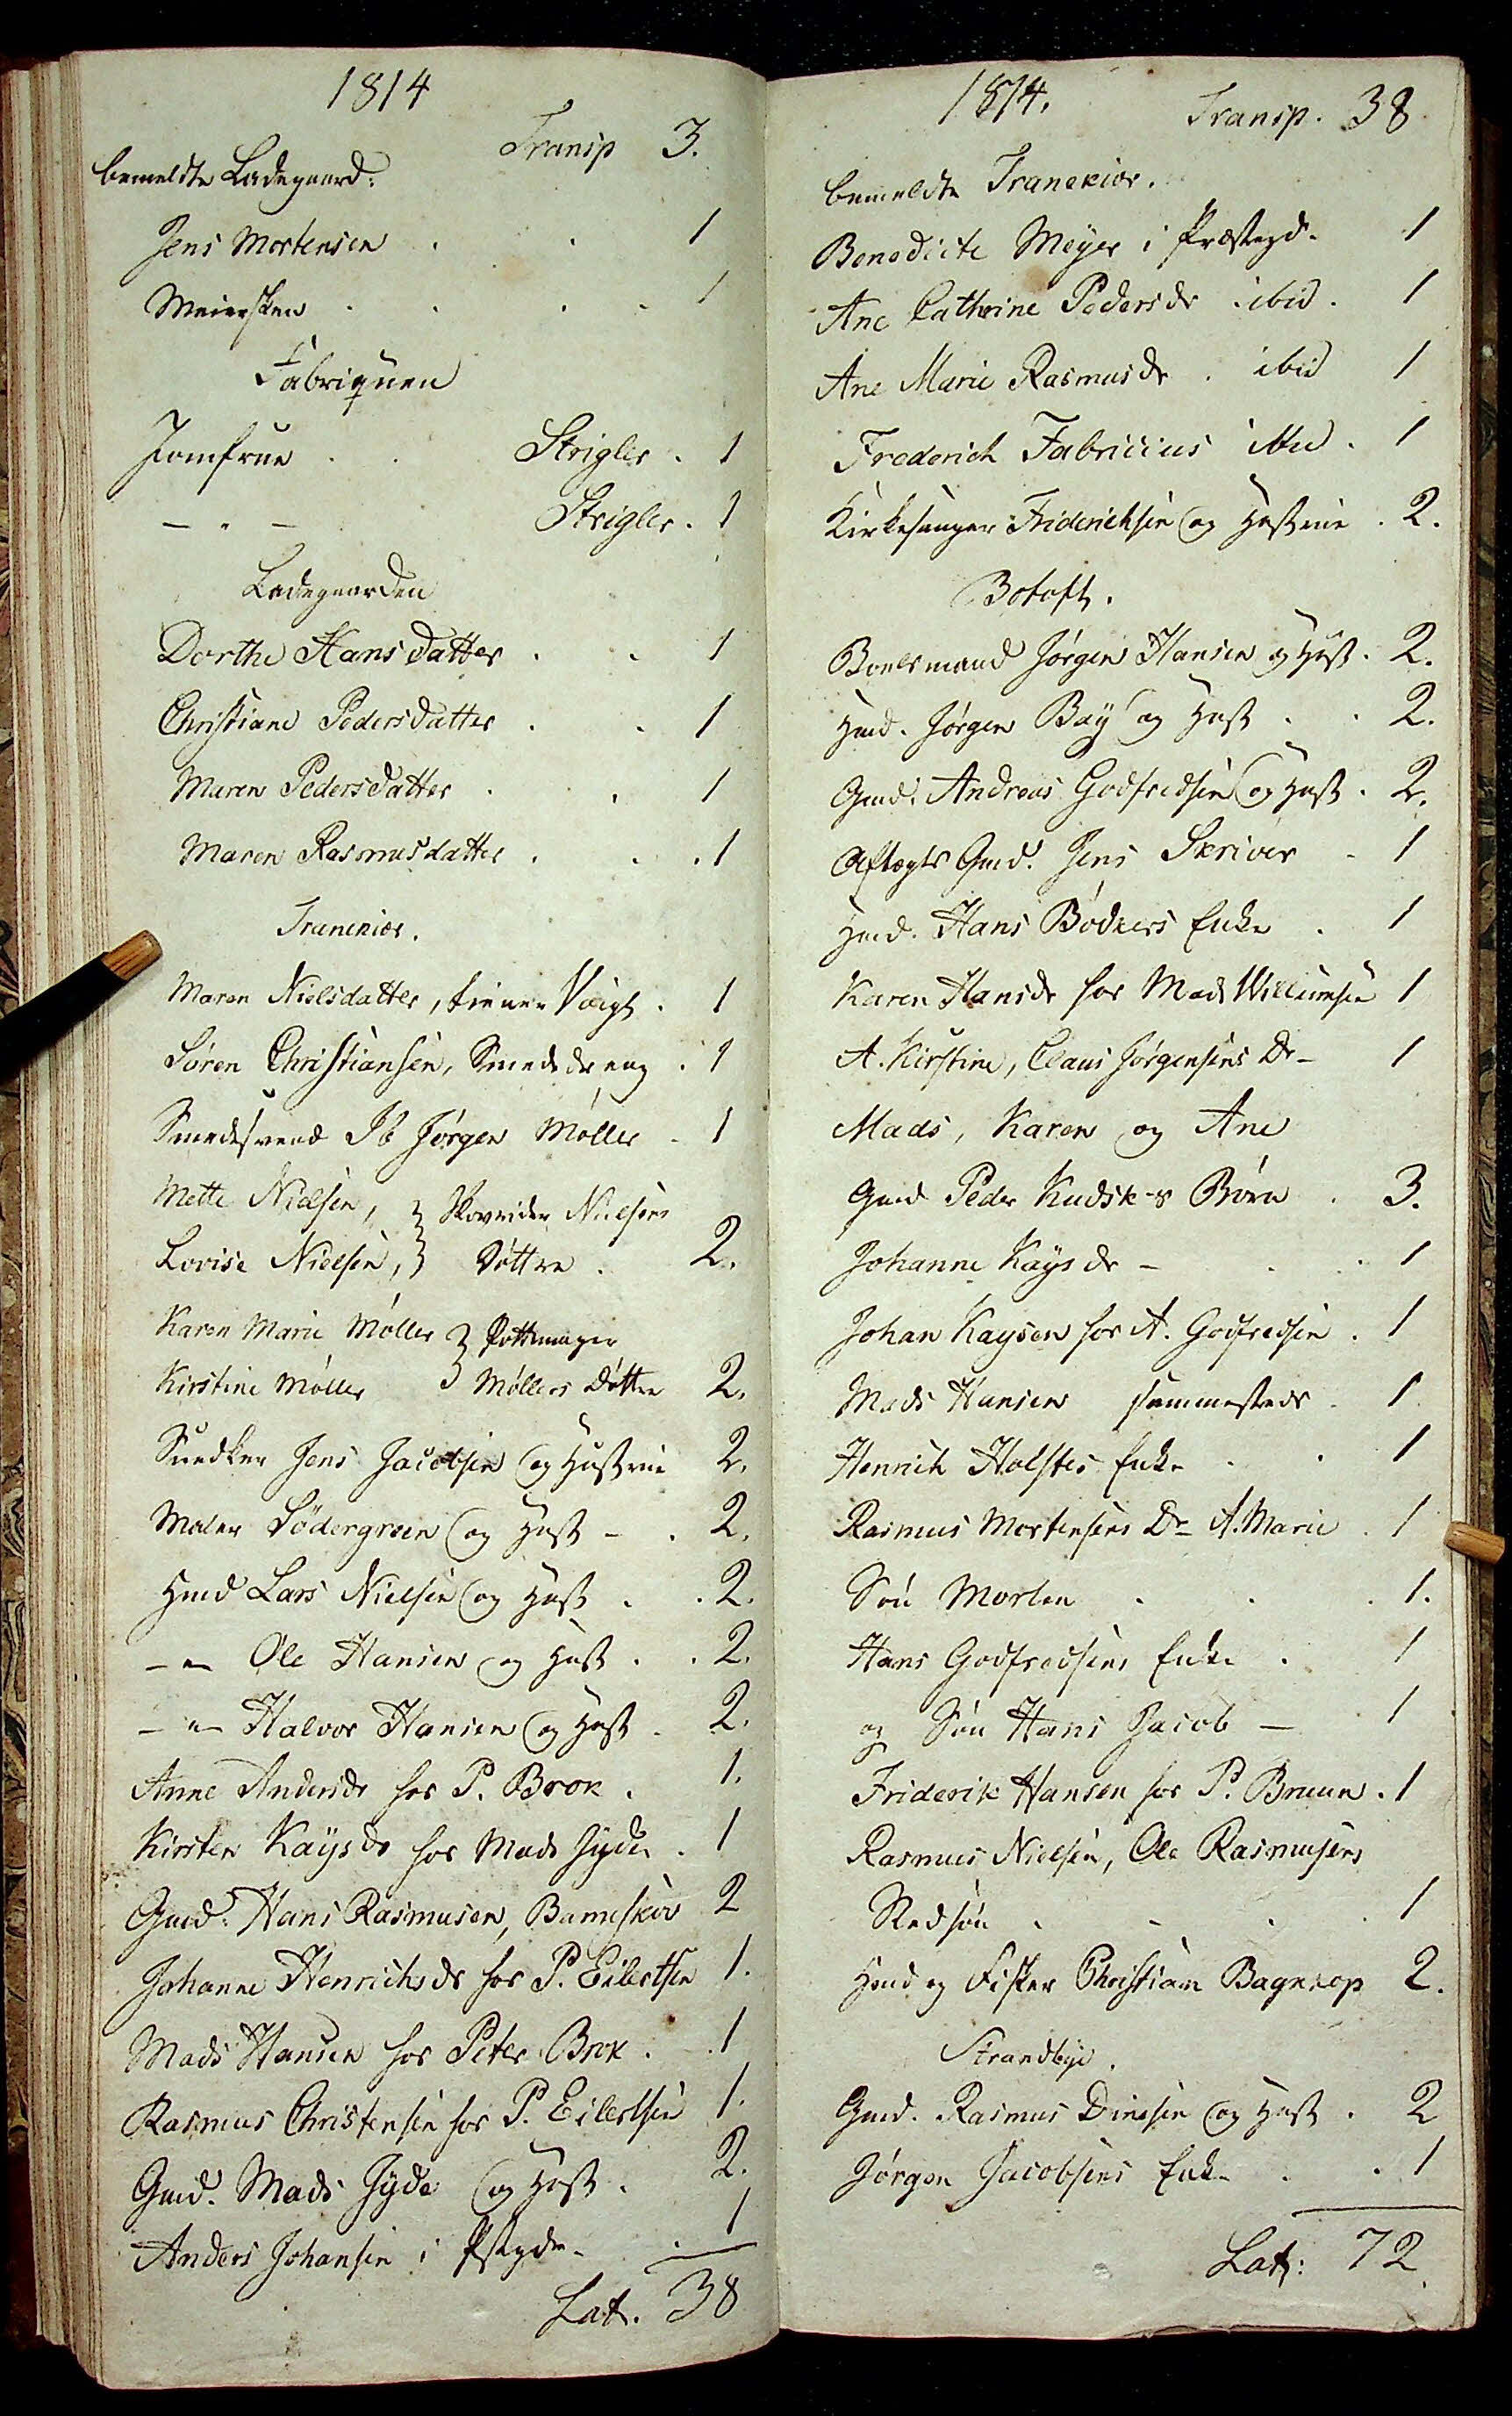1814
Transp 3.
bemeldte Ladegaard:
Jens Mortensen . . 1
Meiersken . . . . 1
Fabriquen
Jomfrue . . Strigler . 1
-"- . Strigler . 1
Ladegaarden
Dorthe Hansdatter . . 1
Christiane Pedersdatter . . 1
Maren Pedersdatter . . . 1
Maren Rasmusdatter . . . 1
Tranekiær
Maren Nielsdatter, tienner Voigt . 1
Søren Christiansen, Smeddreng . 1
Smedsvend Ib Jørgen Møller . 1
Mette Nielsen,
Lovise Nielsen,
Skovrider Nielsens
Døttre . 2.
Karen Marie Møller
Pottemager
Kirstine Møller Møllers Døttre 2.
Snedker Jens Jacobsen og Hustrue 2.
Maler Sødergreen og Hust - . 2.
Hmd Lars Nielsen og Hust . . 2.
-"- Ole Hansen og Hust .  . 2.
-"- Halvor Hansen og Hust . 2.
Anne Andersdr hos P. Brok . 1.
Kirsten Kaysdr hos Mads Jyde . 1
Gmd: Hans Rasmusen Bameskov 2
Johanne Henrichsdr hos P. Eilertsen 1.
Mads Hansen hos Peter Brok . . 1
Rasmus Christensen hos P. Eilertsen 1.
Gud Mads Jyde og Hust . 2
Anders Johansen i Pstgdr . .1
Lat. 38
1814.
Transp. 38
bemeldte Tranekiær.
Bendicte Meyer i Præstedg . 1
Ane Cathrine Pedersdr ibid . 1
Anne Marie Rasmusdr . ibid 1
Frederich Fabricius ibid . 1
Kirkesanger Friderichsen og Hustrue . 2.
Botoft.
Boelsmand Jørgen Hansen og Hust . 2.
Hmd. Jørgen Bay og Hust . . 2.
Gmd Anders Godfredsen og Hust . 2.
Aftægts Gmd. Jens Skriver . 1
Hmd. Hans Bødkers Enke . 1
Karen Hansdr hos Mads Willumsen 1
A. Kirstine, Claus Jørgensens Dr - 1
Mads, Karen og Ane
Gmd Peder Kudsk-s Børn . 3.
Johanne Kaysdr - . . 1
Johan Kaysen hos Godfredsen . 1
Mads Hansen sammesteds . 1
Henrich Holstes Enke . . 1
Rasmus Mortensens Dr A. Marie . 1
Søn Morten . . . 1.
Hans Godfredsens Enke . 1
og Søn Hans Jacob - . 1
Friderik Hansen hos P. Bruun . 1
Rasmus Nielsen, Ole Rasmusens
Stedsøn . . . . 1
Hmd og Fisker Christian Bagnkop 2
Strandbye
Gmd Rasmus Dinesen og Hust . 2
Jørgen Jacobsens Enke . . 1
Lat: 72
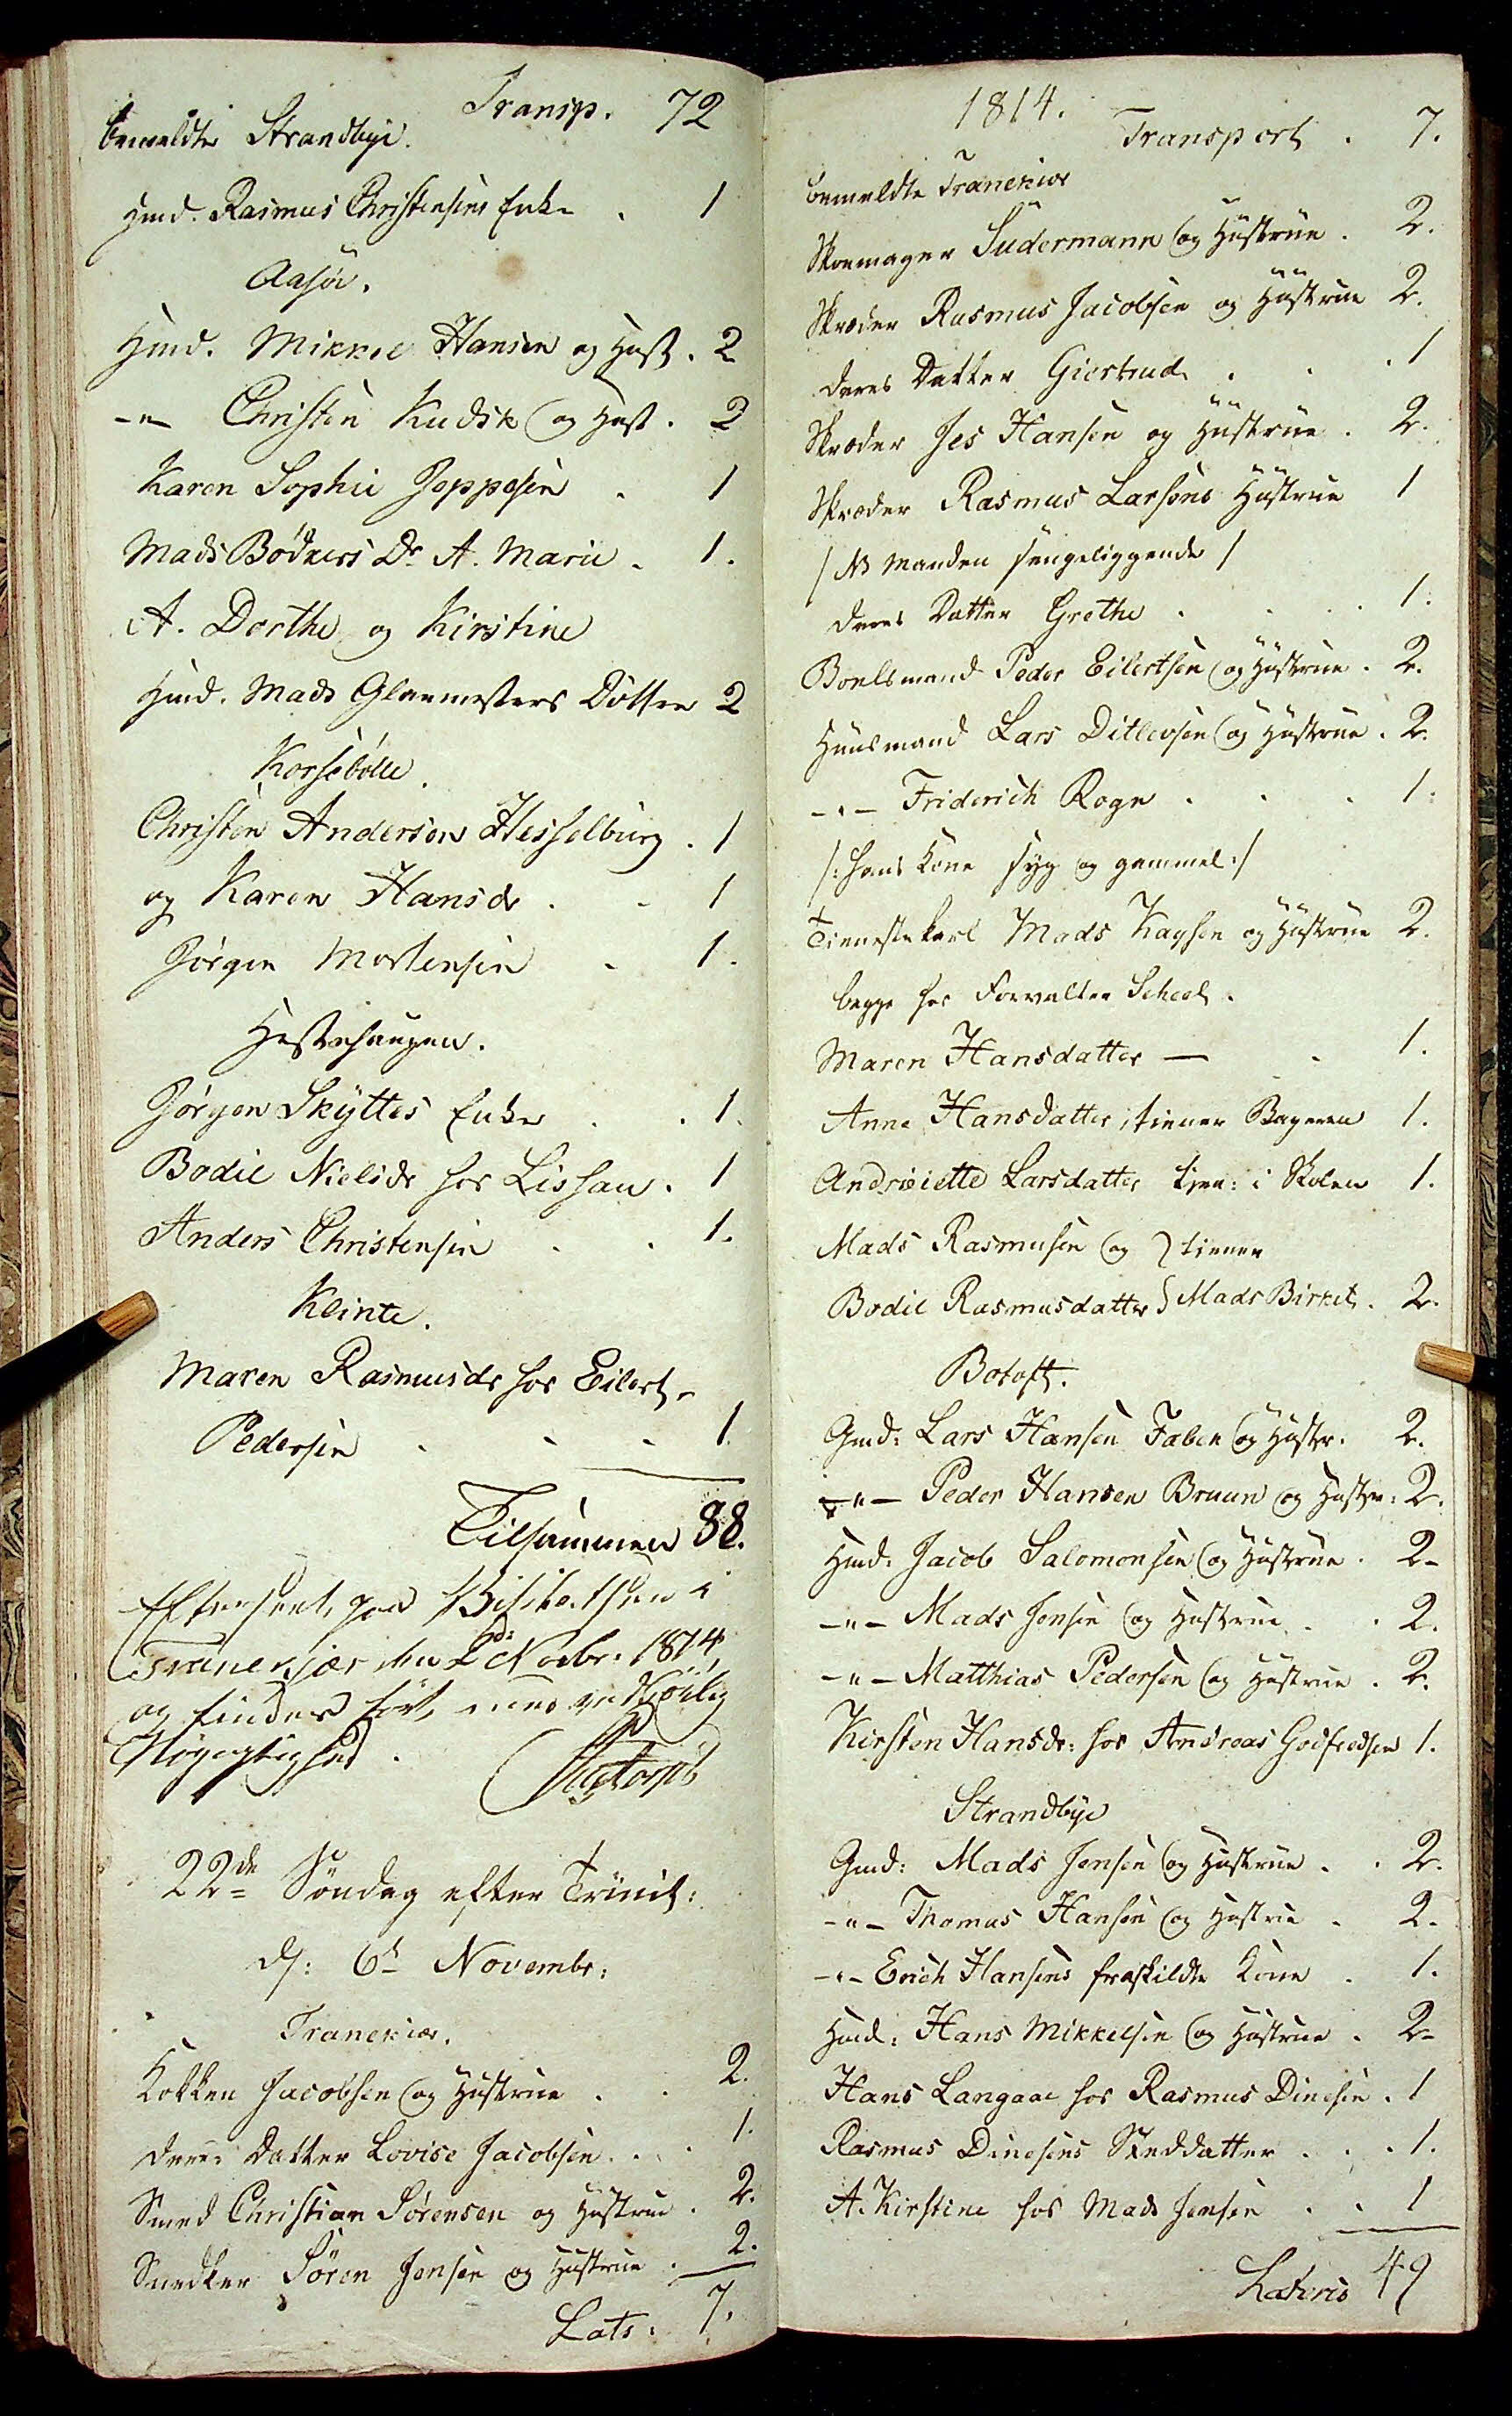Transp. 72
bemeldte Strandbye.
Hmd. Rasmus Christensens Enke . 1
Aasøe.
Hmd. Mikkel Hansen og Hust . 2
-"- Christen Kudsk og Hust . 2
Karen Sophie Jeppesen .1
Mads Bødkers Dr A. Marie . 1.
A. Dorthe og Kirstine
Hmd. Mads Glarmesters Datter 2
Korsebølle
Christen Andersen Hesselbierg . 1
og Karen Hansdr . . 1
Jørgen Mortensen . 1.
Hestehaugen.
Jørgen Skyttes Enke . . 1
Bodil Nielsdr hos Lissau . 1
Anders Christensen . . 1.
Klinte.
Maren Rasmusdr hos Eiler -
Pedersen . . . 1.
Tilsammen 88.
Efterseet paa Visitatten i
Tranerkjær den 2de Novbr. 1814
og findes ført med ## ##
Nøjagtighed
##
22de Søndag efter Trinit:
d: 6te Novembr:
Tranekiær.
Kokken Jacobsen og Hustrue . . 2.
deres Datter Lovise Jacobsen . . . . 1.
Smed Christian Sørensen og Hustrue . 2.
Snedker Søren Jensen og Hustrue . 2.
Lats: 7
1814.
Transport. 7.
bemeldte Tranekiær.
Skoemager Sudermann og Hustrue . 2.
Skræder Rasmus Jacobsen og Hustrue 2.
deres Datter Giertrud . . . 1
Skræder Jes Hansen og Hustrue . 2.
Skræder Rasmus Larsens Hustrue 1
/ NB Manden sengeliggende /
deres Datter Grethe . . . . 1.
Boelsmand Peder Eilertsen og Hustrue  . 2.
Huusmand Lars Ditlevsen og Hustrue . 2.
-"- Friderich Rogn . . . 1.
/: Hans Kone syg og gammel :/
Tienestekarl Mads Kaysen og Hustrue 2.
begge hos Forvalter Scheel.
Maren Hansdatter - . 1.
Anne Hansdatter, tiener Bageren 1.
Andrieiette Larsdatter tien: i Skolen 1.
Mads Rasmusen og
Bodil Rasmusdatter
tienen
Mads Birket . 2.
Botoft.
Gmd: Lars Hansen Fæbek og Hustr . 2.
-"- Peder Hansen Bruun og Hustru : 2
Hmd: Jacob Salomonsen og Hustrue . 2.
-"- Mads Jensen og Hustrue . . 2.
-"- Matthias Pedersen og Hustrue . 2.
Kirsten Hansdr: hos Andreas Godfredsen 1.
Strandbye
Gmd: Mads Jensen og Hustrue . . 2.
-"- Thomas Hansen og Hustrue . 2.
-"- Erich Hansens fraskildte Kone . 1.
Hmd: Hans Mikkelsen og Hustrue . 2-
Hans Langaae hos Rasmus Dinesen . 1
Rasmus Dinesens Steddatter . . . 1.
A. Kirstine hos Mads Jensen . . 1
Lateris 49